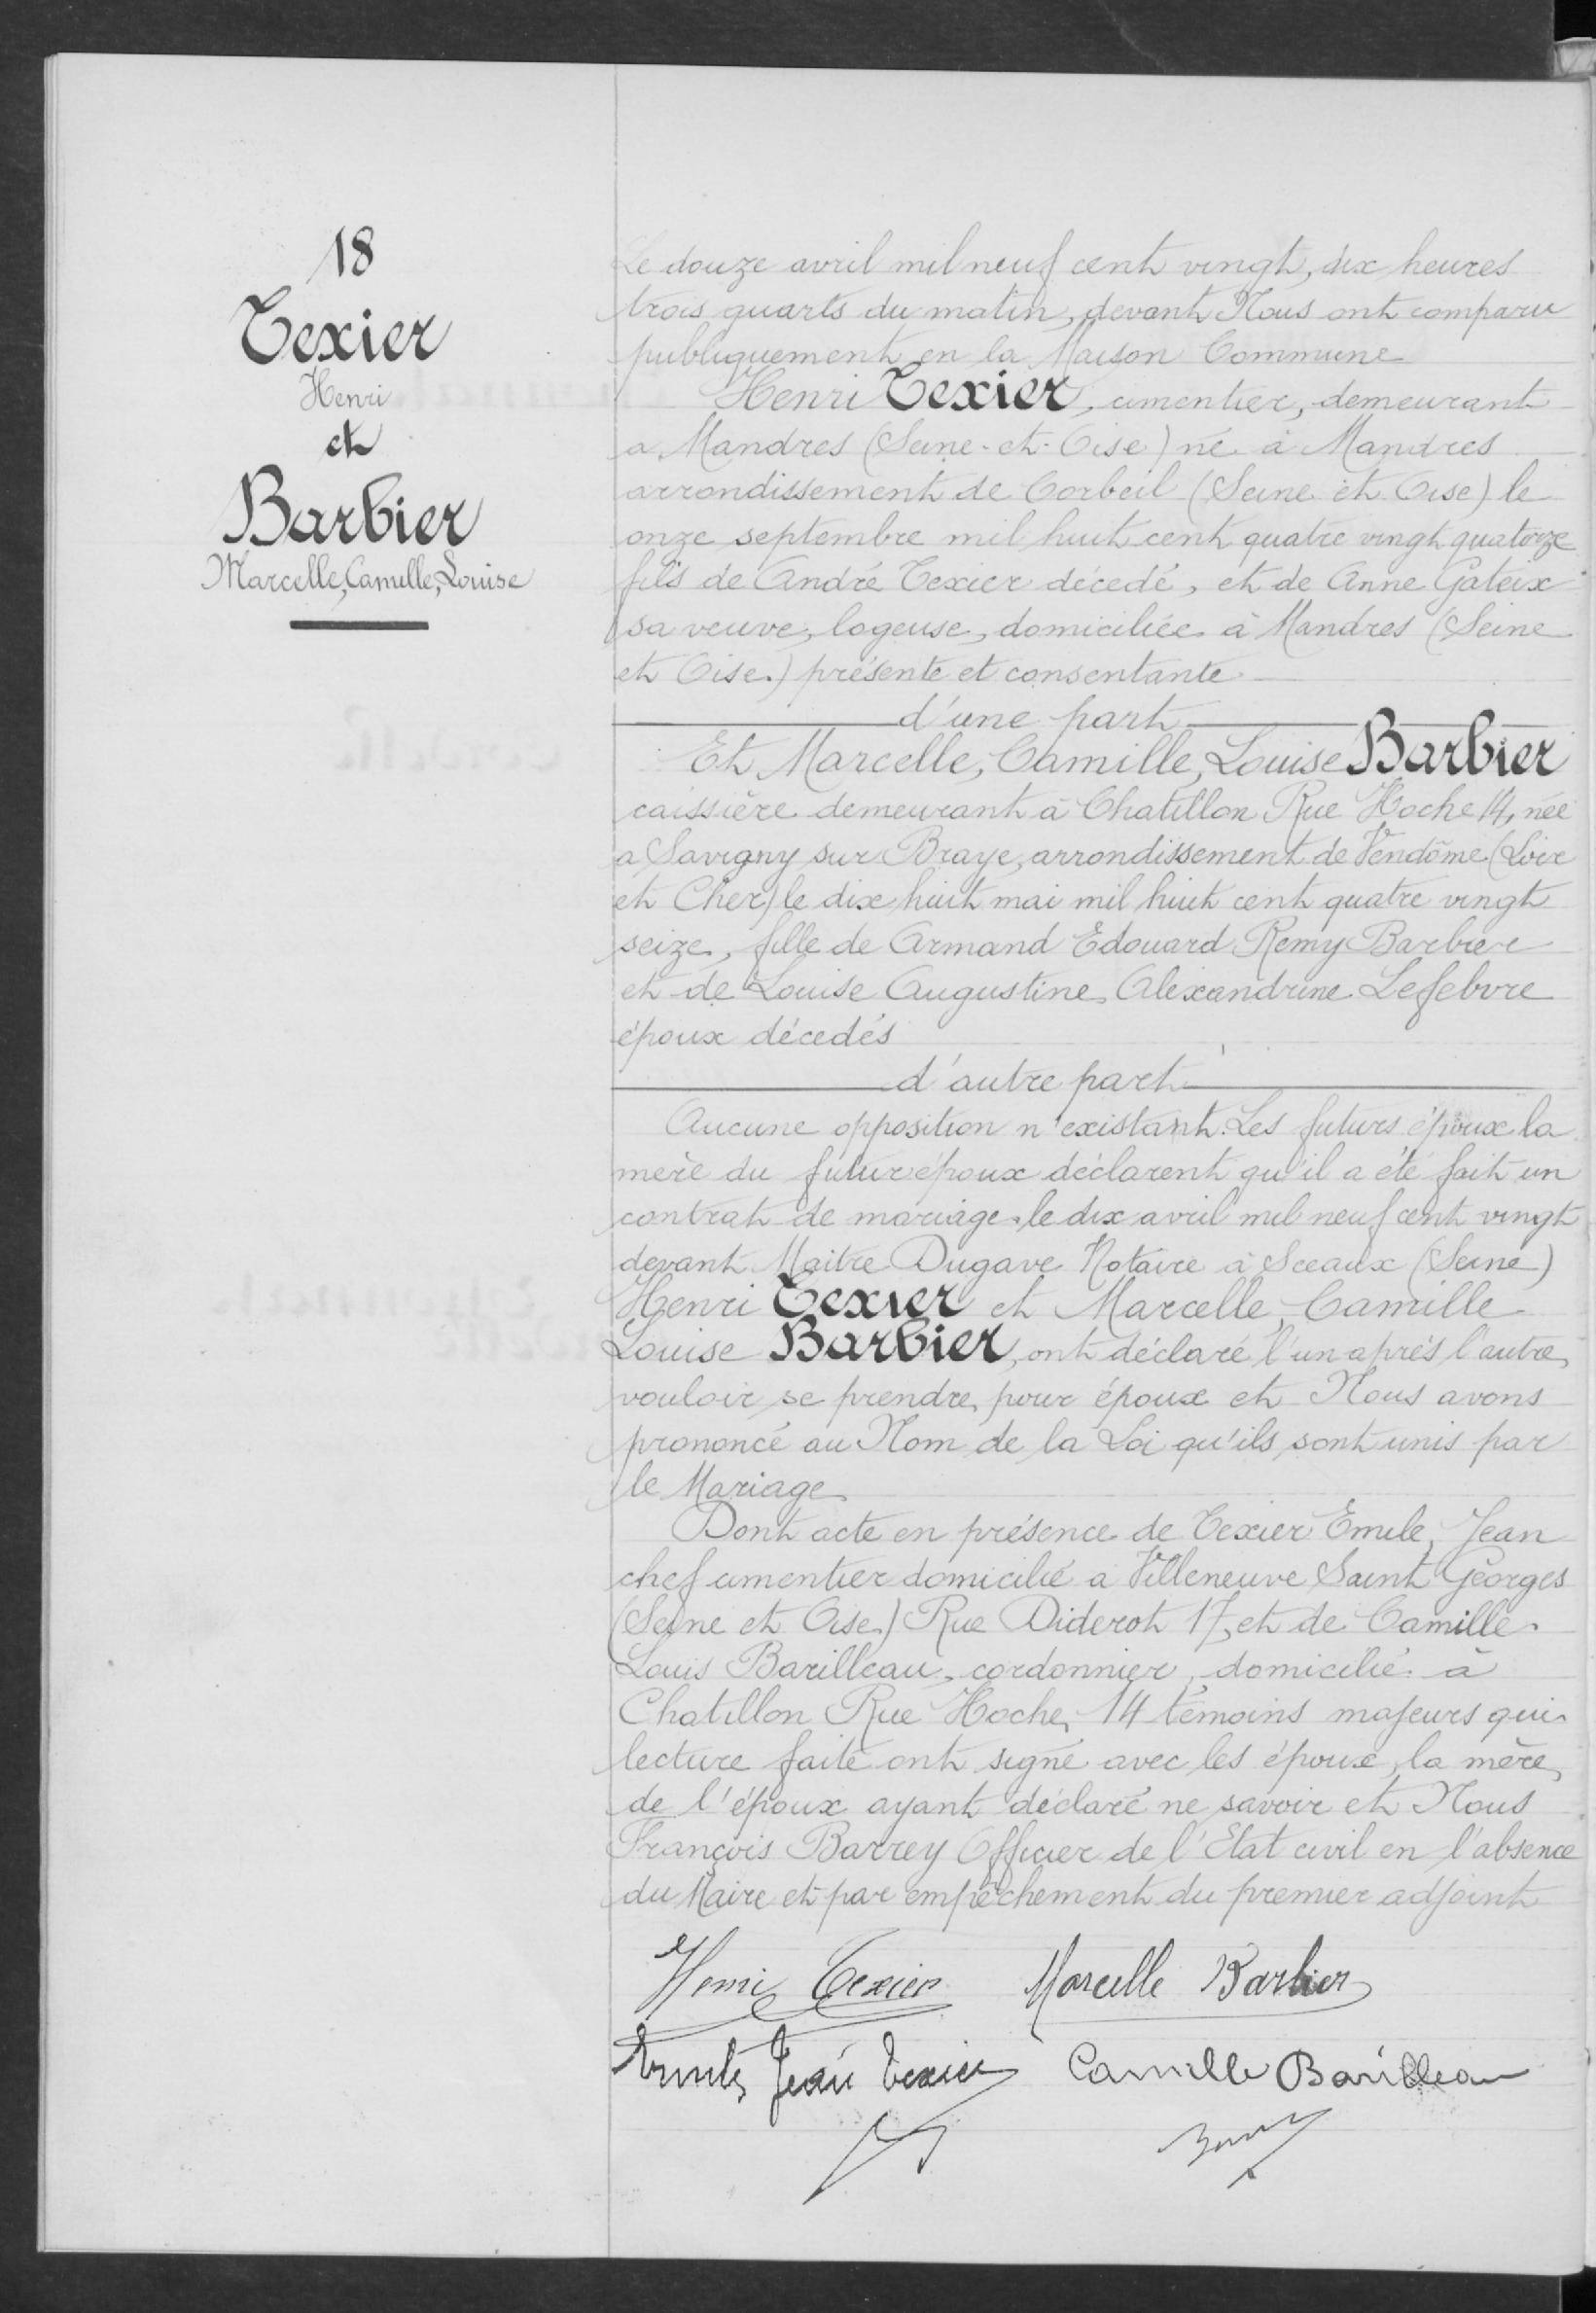18
Texier
Henri
et
Barbier
Marcelle, Camille, Louise
Le douze avril mil neuf cent vingt, dix heures
trois quarts du matin, devant Nous ont comparu
publiquement en la Maison Commune
Henri Texier, cimentier, demeurant
a Mandres (Seine-et-Oise) né à Mandres
arrondissement de Corbeil (Seine et Oise) le
onze septembre mil huit cent quatre vingt quatorze
fils de André Texier décédé, et de Anne Gateux
sa veuve, logeuse, domiciliée à Mandres (Seine
et Oise) présente et consentante
-d'une part-
Et Marcelle, Camille, Louise Barbier
caissière demeurant à Chatillon Rue Hoche 14, née
a Savigny sur Braye, arrondissement de Vendôme (Loire
et Cher) le dix huit mai mil huit cent quatre vingt
seize, fille de Armand Edouad Remy Barbiere
et de Louis Augustine, Alexandrine Lefebvre
époux décédés
-d'autre part-
Aucune opposition n'existant. Les futurs poux la
mère du futur époux déclarent qu'il a été fait un
contrat de mariage le dix avril mil neuf cent vingt
devant Maitre Dugave Notaire à Sceaux (Seine)
Henri Texier et Marcelle Camille
Louise Barbier ont déclaré l'un après l'autre,
vouloir se prendre pour époux et Nous avons
prononcé au Nom de la Loi qu'ils sont unis par
le Mariage
Dont acte en présence de Texier Emile, Jean
chef cimentier domicilié à Villeneuve Saint Georges
(Seine et Oise) Rue Diderot 17, et de Camille
Louis Barilleau, cordonnier domicilié à
Chatillon Rue Hoche, 14 témoins majeurs qui
lecture faite ont signé avec les époux, la mère
de l'époux ayant déclaré ne savoir et Nous
François Barrey Officier de l'Etat civil en l'absence
du Maire et par empêchement du premier adjoint
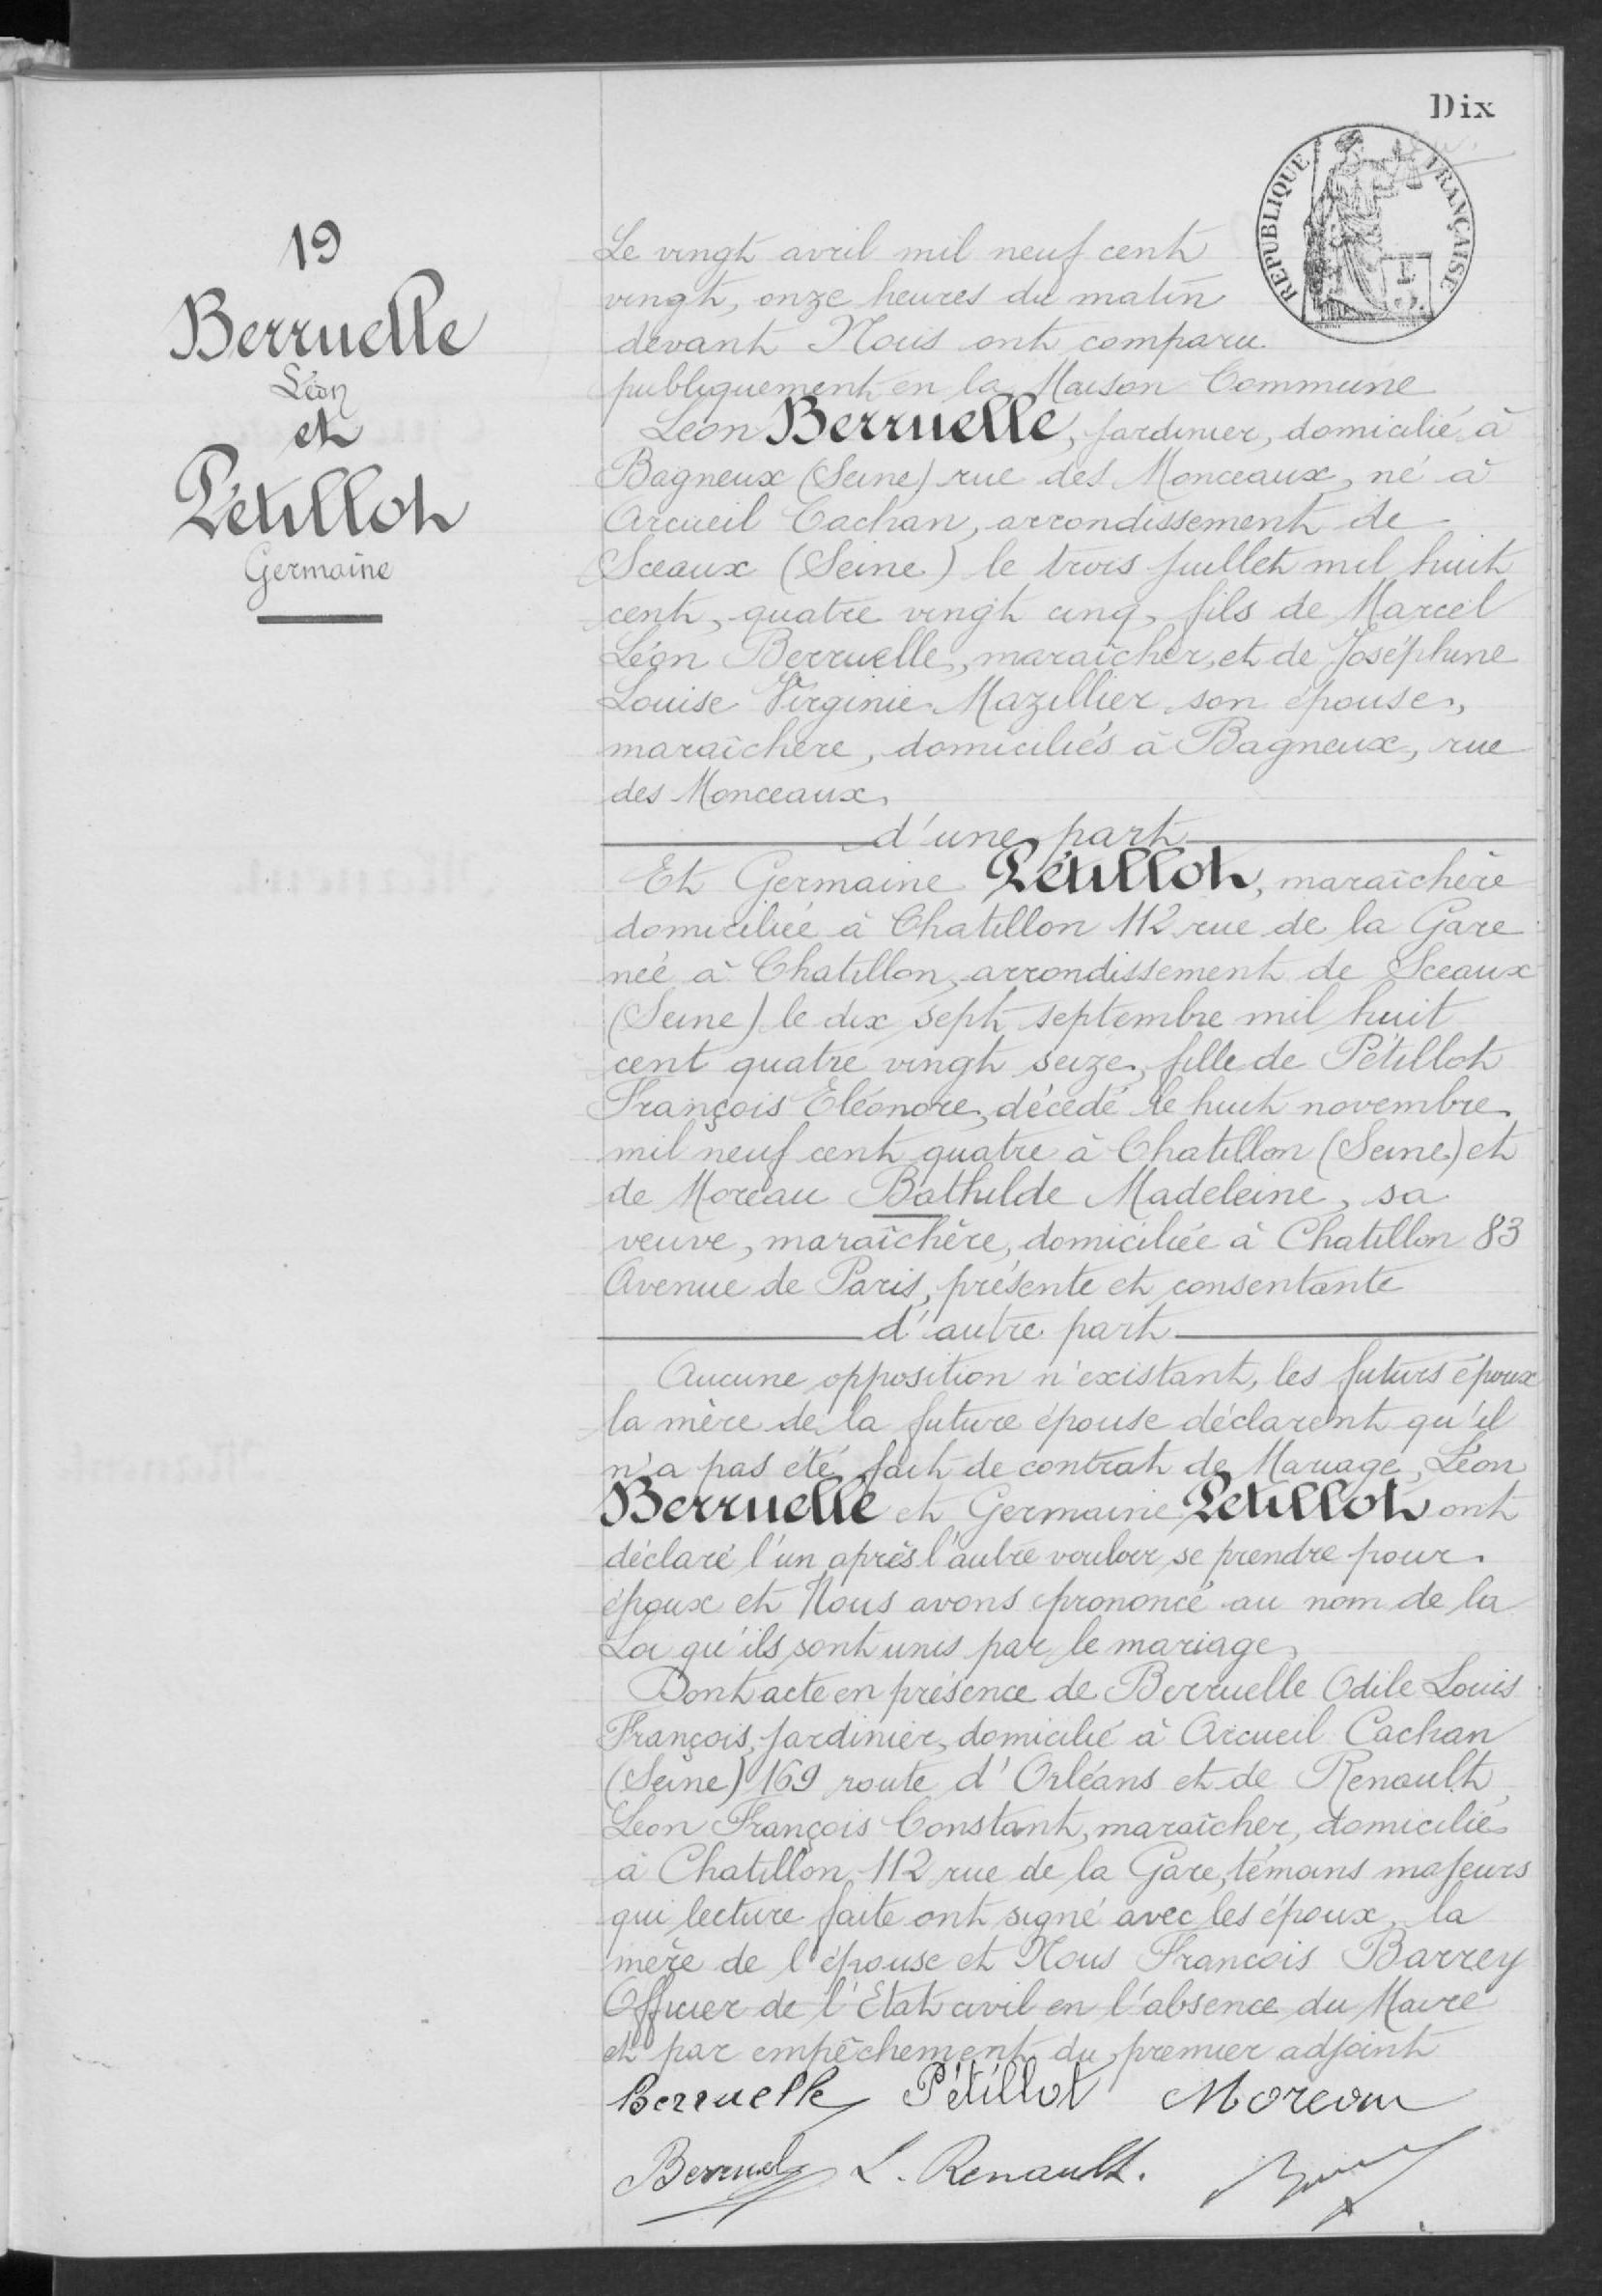19
Berruelle
Léon
et
Pétillon
Germaine
Le vingt avril mil neuf cent
vingt, onze heures du matin
devant Nous ont comparu
publiquement en la Maison Commune
Léon Berruelle, jardinier, domicilié à
Bagneux (Seine) rue des Monceaux, né à
Arcueil Cachan, arrondissement de
Sceaux (Seine) le trois juillet mil huit
cent, quatre vingt cinq, fils de Marcel
Léon Berruelle, maraîcher et de Joséphine
Louise Virginie Mazillier son épouse,
maraîchère, domiciliés à Bagneux, rue
des Monceaux
-d'une part-
Et Germaine Létullot, maraîchère
domiciliée à Chatillon 112 rue de la Gare
ne à Chatillon, arrondissement de Sceaux
(Seine) le dix sept septembre mil huit
cent quatre vingt seize, fille de Petilllot
Françoise Eléonore, décédé le huit novembre
mil neuf cent quatre à Chatillon (Seine) et
de Moreau Bathilde Madeleine, sa
veuve, maraîchère, domiciliée à Chatillon 83
Avenue de Paris, présente et consentante
-d'autre part -
Aucune opposition n'existant, les futurs époux
la mère de la future épouse déclarent qu'il
n'a pas été fait de contrat de Mariage, Léon
Berruelle et Germaine Petillot ont
déclaré l'un après l'autre vouloir se prendre pour
époux et Nous avons prononcé au nom de la
Loi qu'ils sont unis par le mariage
Dont l'acte en présence de Berruelle Odile Louis
François, jardinier, domicilié à Arcueil Cachan
(Seineà 169 route d'Orléans et de Renault
Léon François Constant, maraîcher, domicilié
à Chatillon 11é rue de la Gare, témoins majeurs
qui lecture faite ont signé avec les époux, la
mère de l'épouse et Nous François Barrey
Officier de l'Etat civil en l'absence du Maire
et par empêchement du premier adjoint